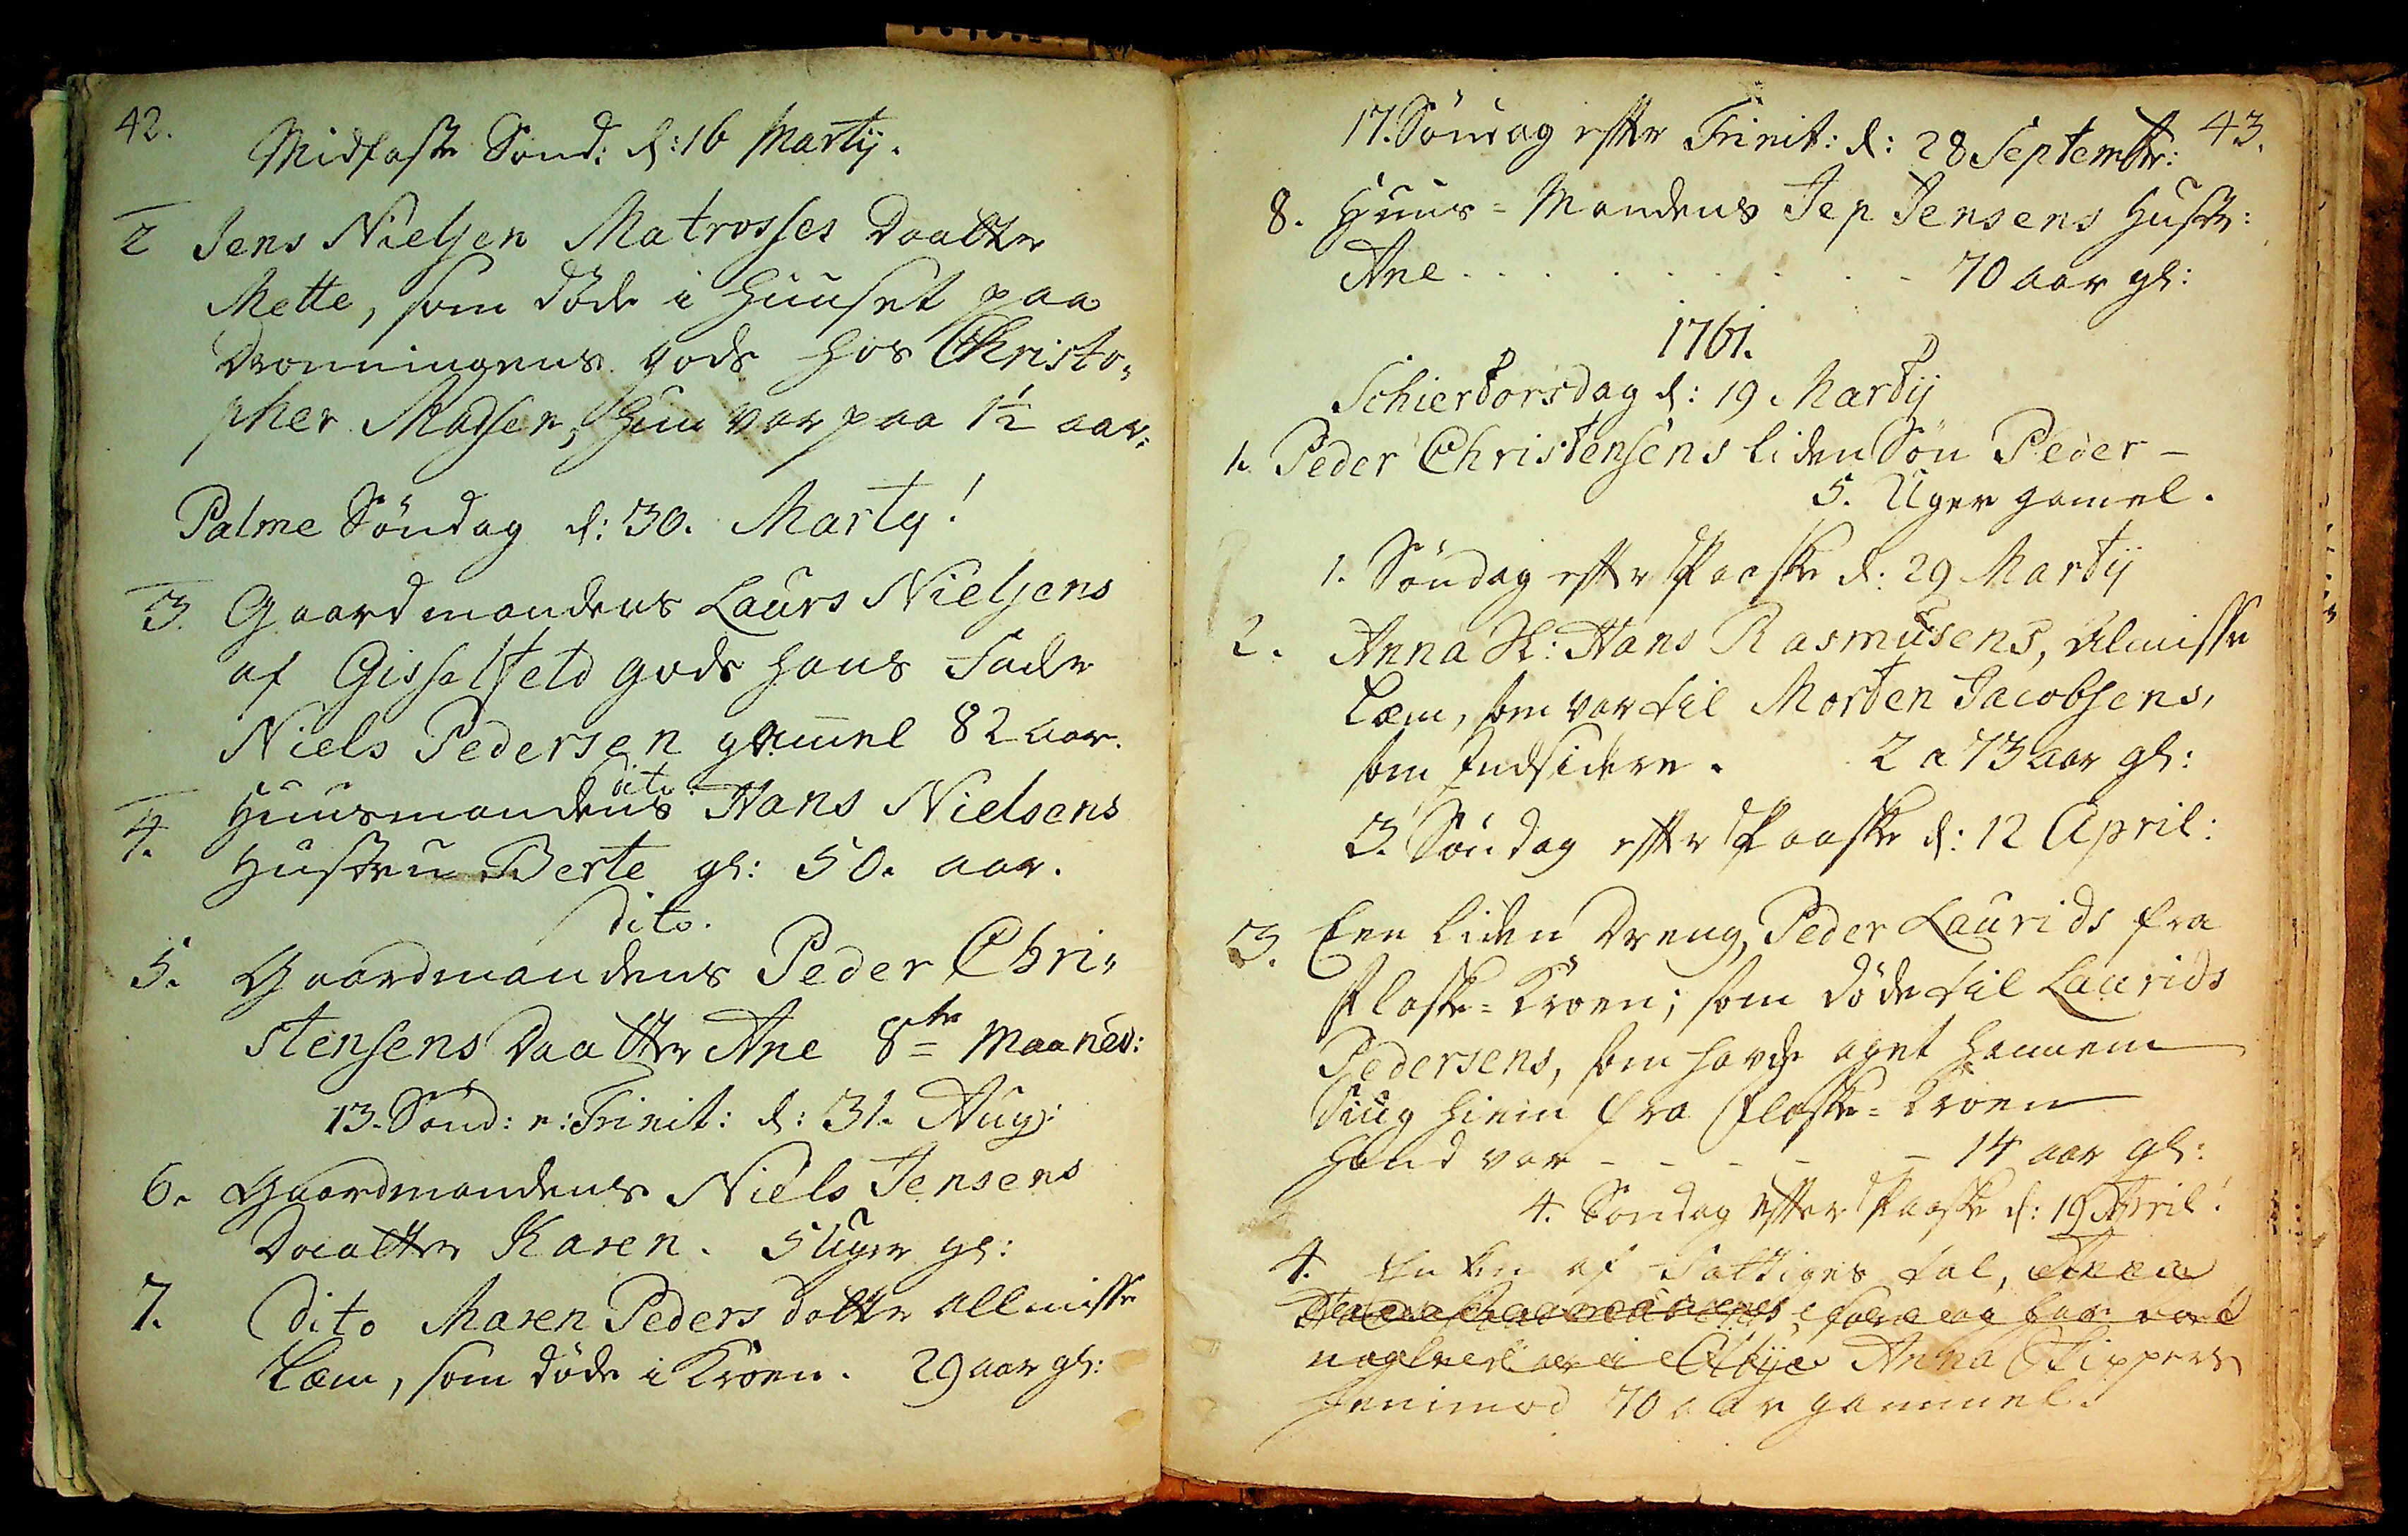42.
Midfaste Sønd: d: 16 Martij.
2. Jens Nielsen Matrosses Daatter
Mette, som døde i Huuset paa
Dronningens Gods hos Christo¬
pher Madsen, Hun var paa 1½ aar.
Palme Søndag d: 30. Martij !
3. Gaardmandens Laurs Nielsens
af Gisselfeld Gods hans Fade
Niels Pedersen gammel 82 aar.
dito.
4. Huusmandens Hans Nielsens
Hustru Berte gl: 50. aar.
dito.
5. Gaardmandens Peder Chri¬
stensens Daatter Ane 8te Maaned:
13. Sønd: e: Trinit: d: 31. Aug:
6. Gaardmandens Niels Jensens
Daatter Karen. 5 Uger gl:
7. dito Maren Peders dotter Allmisse
Læm, som døde i Kroen. 29 aar gl:
43.
17. Søndag efter Trinit: d: 28 Septembr:
8. Huus = Mandens Jep Jensens Hustr:
Ane  
70 aar gl:
1761.
Schiertorsdag d: 19 Martij
1. Peder Christensens liden Søn Peder -
5. Uger gammel.
1. Søndag efter Paaske d: 29 Martij
2. Anna Sl: Hans Rasmussens, Almisse
Læm, som var til Morten Jacobsens,
som Indsidere. 2 a 73 aar gl:
3. Søndag efter Paaske d: 12 April:
3. Een liden Dreng, Peder Laurids fra
Flaske = Kroen; som døde til Laurids
Pedersens, som havde aget Hannem
Siug Hiem fra Flaske = Kroen
Hand var 
14 aar gl:
4. Søndag efter Paaske d: 19 April!
4. Enken af Fattiges tal, Anna
Skippers##, som og har boet
nogle Aar i Ølbye Anna Skippers
henimod 70 aar gammel.
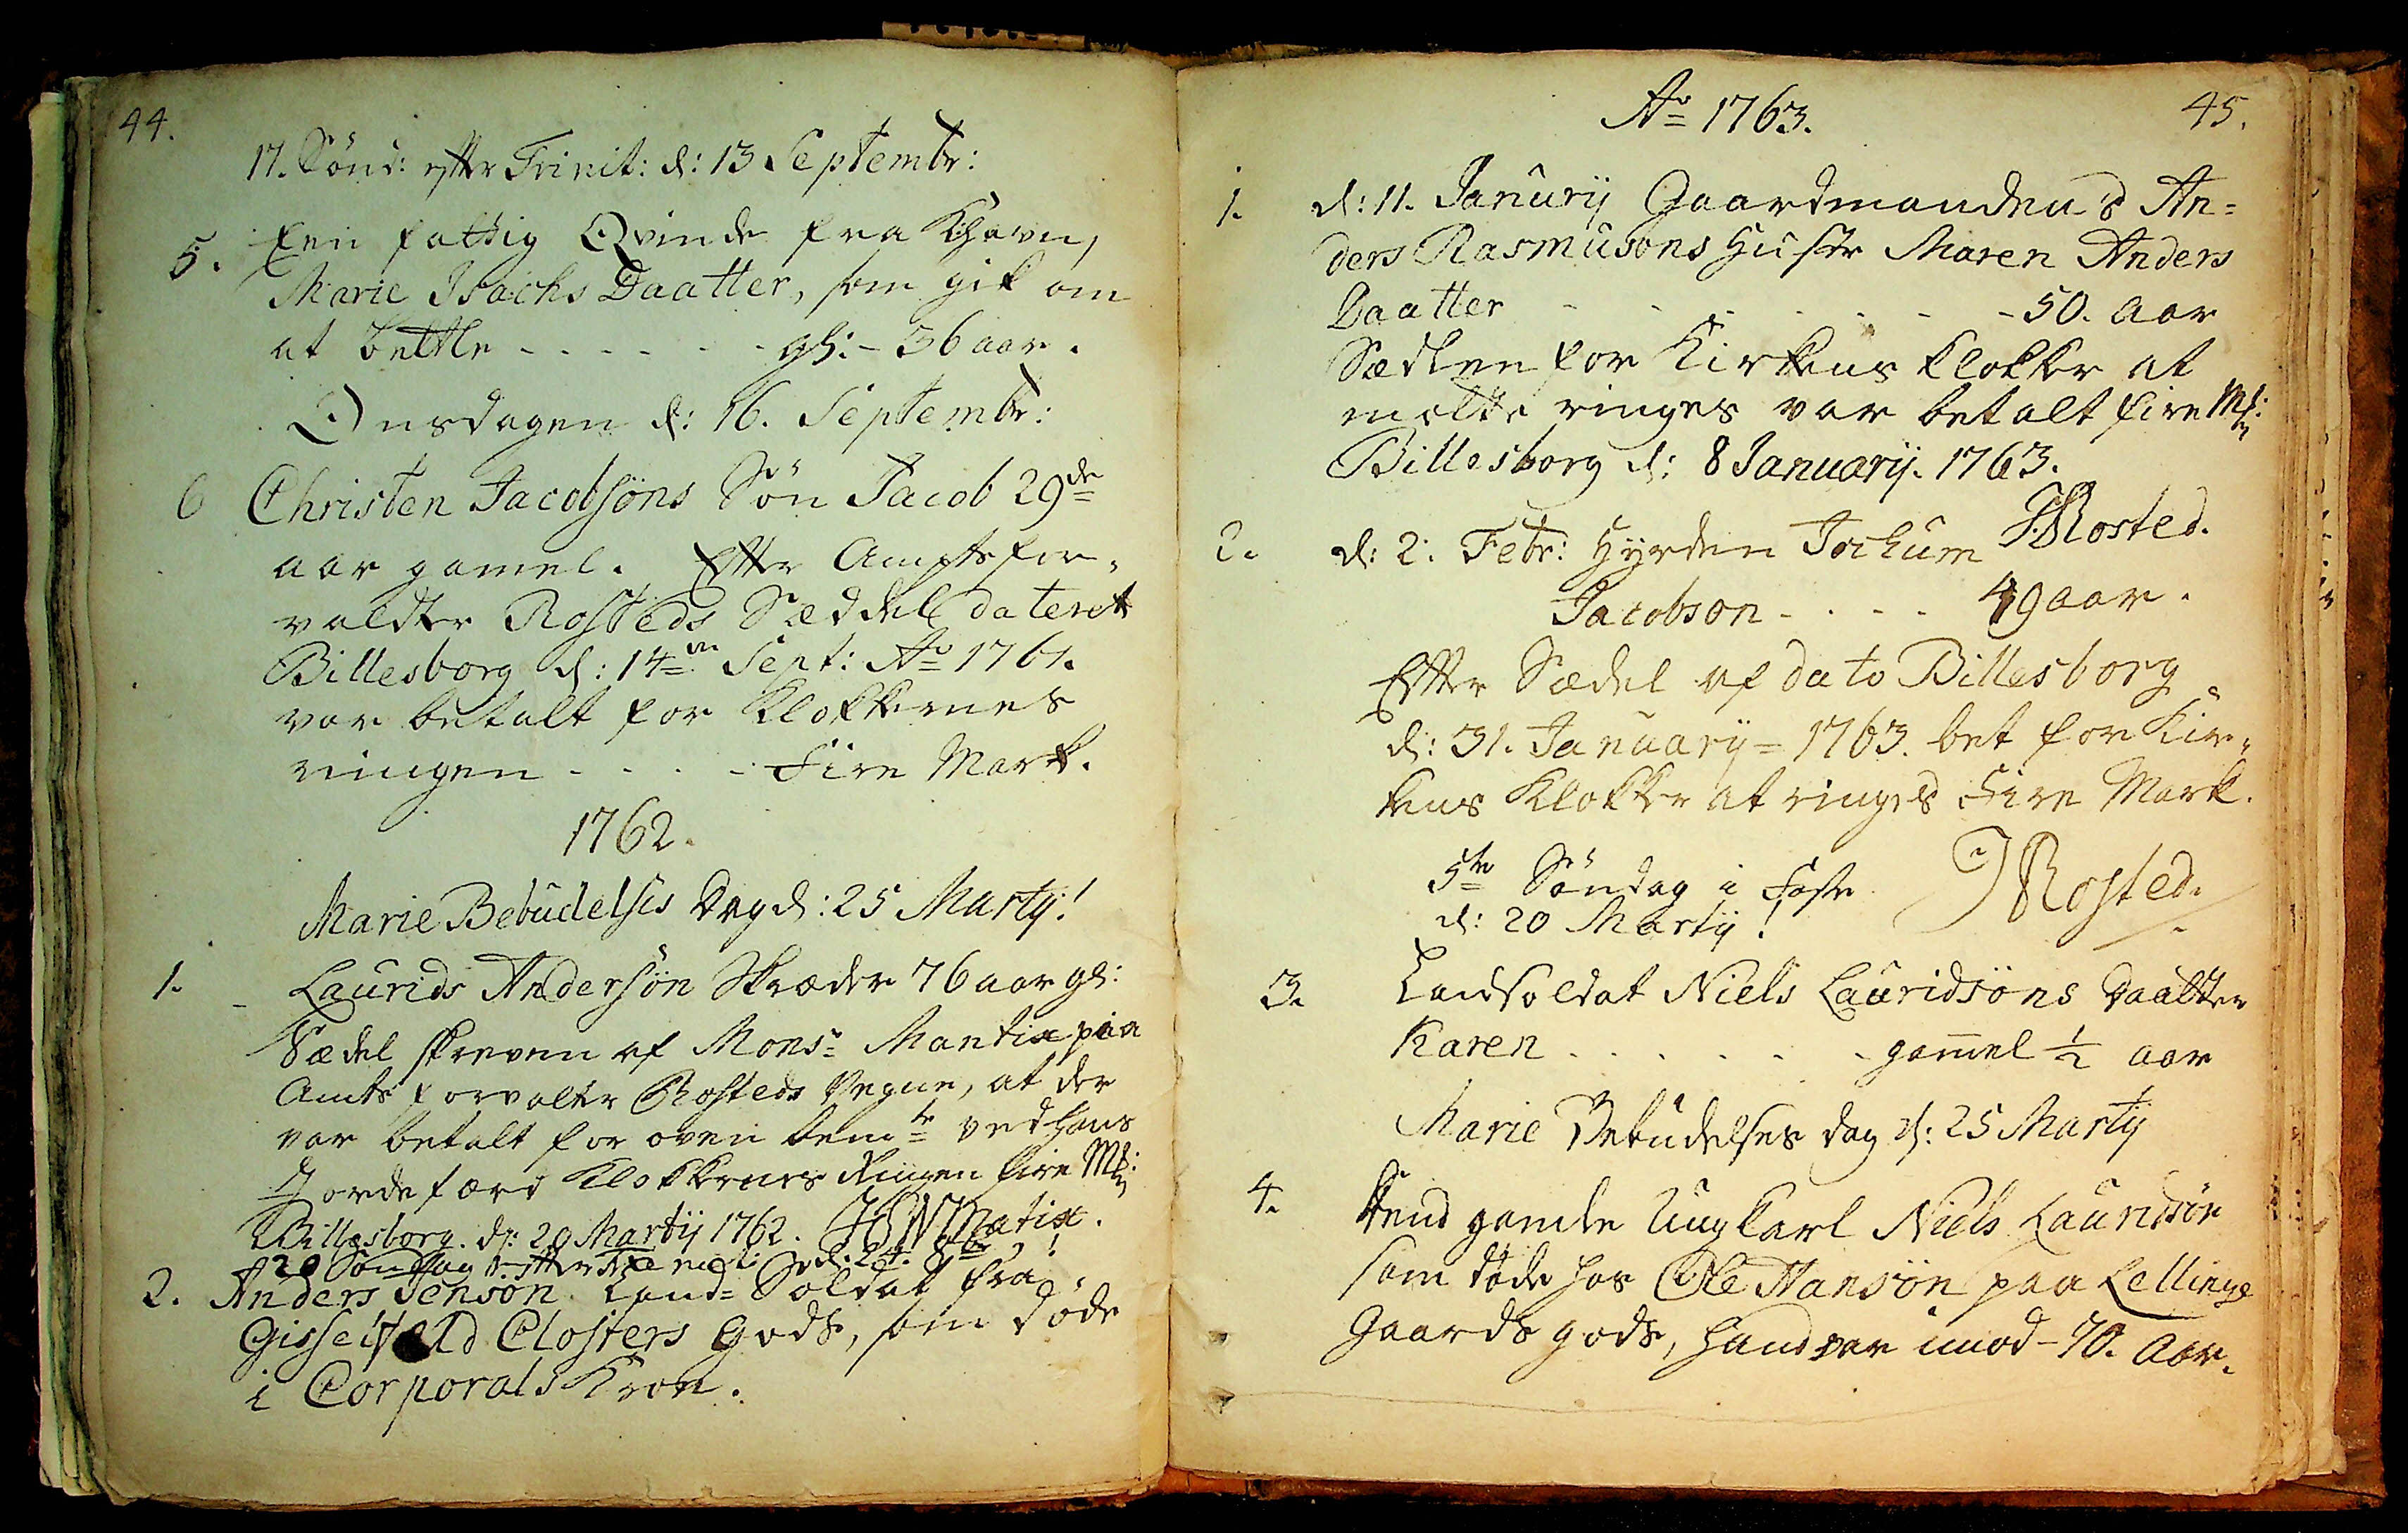44.
17. Sønd: efter Trinit: d: 13 Septembr:
5. Een fattig Qvinde fra K:havn,
Marie Isachs Daatter, som gik om
at bettle 
gl: - 36 aar.
Onsdagen d: 16. Septembr:
6. Christen Jacobsøns Søn Jacob 29de
aar gammel. Efter Amptsfor¬
valdter Rosted Sæddel dateret
Billesborg d: 14de Sept: Ao 1761.
var betalt for Klokkernes
ringen . . . . Fire Mark
1762.
Marie Bebudelses Dag d: 25 Martij!
1. Laurids Andersøn Skræder 76 aar gl:
Sædel skreven af Monsr Mantix paa
Amtsforvalter Rosteds Vegne, at der
var betalt for oven bemte ved Hans
Jordefærd Klokkernes Ringen Fire Mk:
Billesborg. d: 20 Martij 1762. HSWMatix.
20 Søndag efter Trinit: d. 24. 8br !
2. Anders Jensøn Land = Soldat fra
Gisselfeld Closters Gods, som døde
i Corporals Kroe.
45.
Ao 1763.
1. d: 11. Januarij Gaardmandens An¬
ders Rasmusøns Hustr Maren Anders
Daatter 
50. aar
Sædlen for Kirkens Klokker at
motte ringes var betalt fire Mk:
Billesborg d: 8 Januarij. 1763.
J.Rosted.
2. d: 2. Febr: Hyrden Jochum
Jacobson . . . . 49 aar.
Efter Sædel af dato Billesborg.
d: 31. Januarij = 1763. bet for Kir¬
kens Klokker at ringes Fire Mark.
5te Søndag i Faste
J Rosted
d: 20 Martij!
3. Landsoldat Niels Lauridsøns Daatter
Karen
gammel ½ aar
Marie Bebudelses dag d: 25 Martij
4. Dend gamle Ungkarl Niels Lauridsøn
som døde hos Ole Hansøn paa Lellinge
Gaards Gods, Hand var imod - 70. Aar.